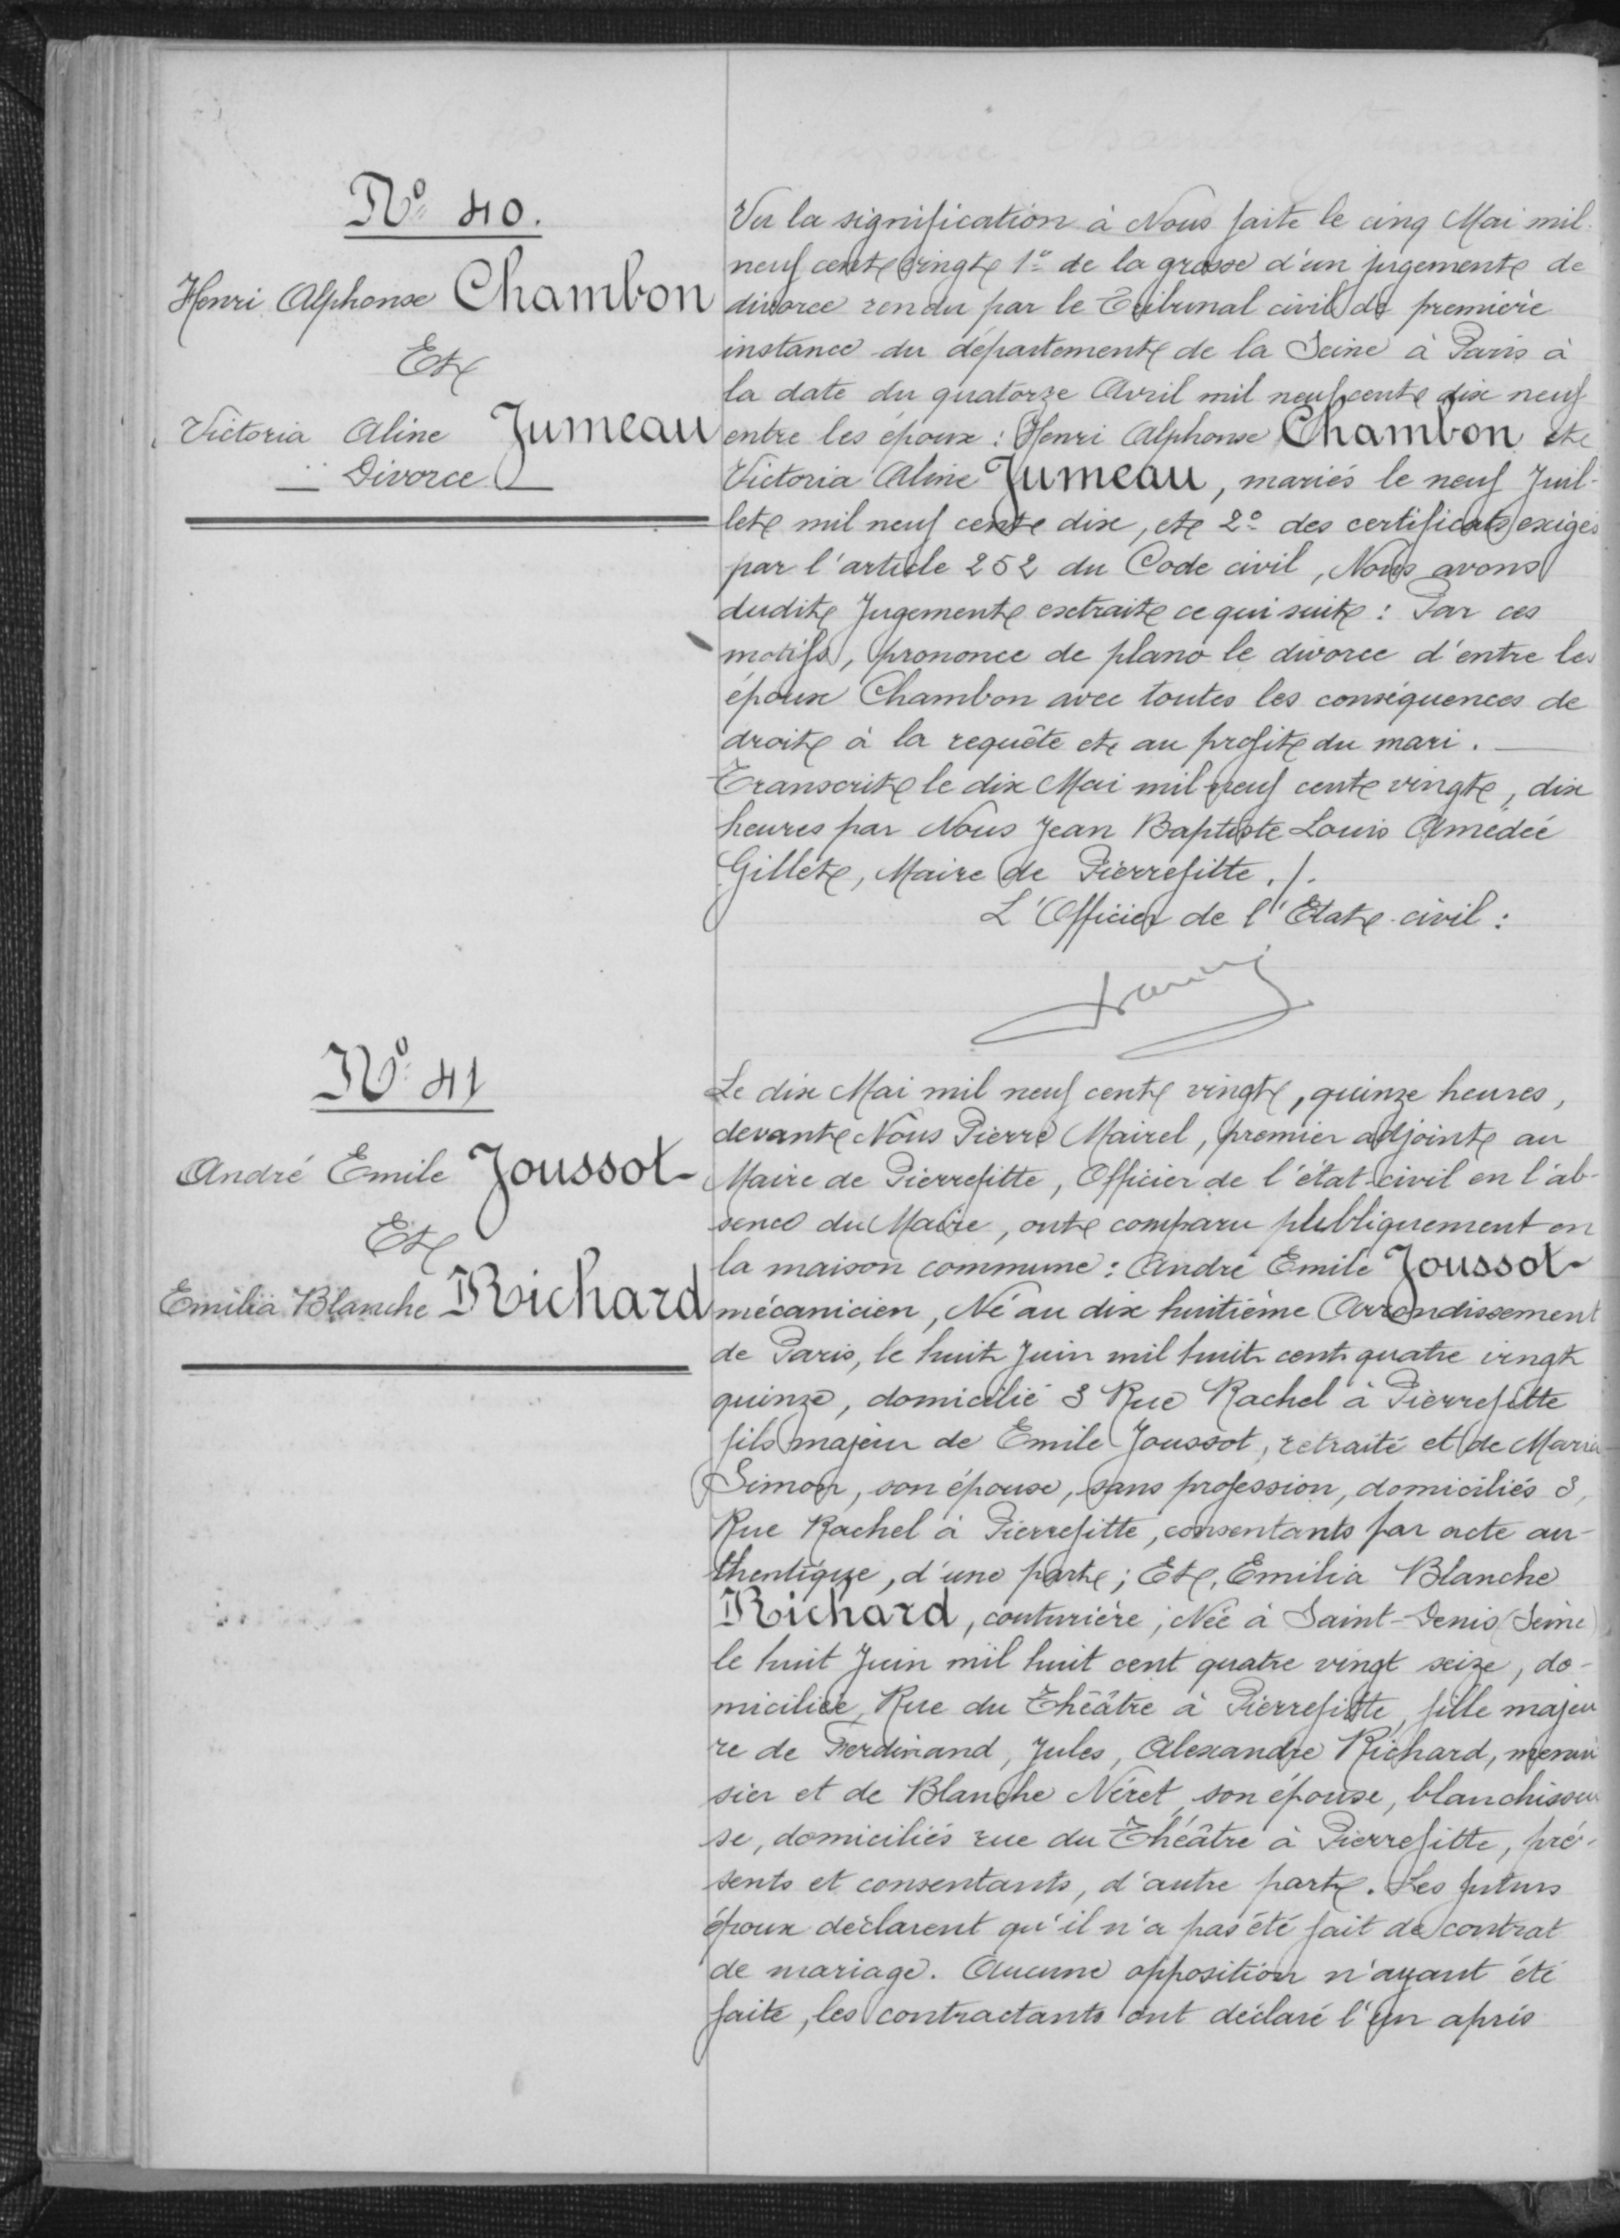N°40
Henri Alphonse Chambon
Et
Victoria Aline Jumeau
Divorce
Vu la signification à Nous faite le cinq Mai mil
neuf cent vingt 1° de la grosse d'un jugement de
divorce rendu par le Tribunal civil de première
instance du département de la Seine à Paris à
la date du quatorze Avril mil neuf cent dix neuf
entre les époux: Henri Alphonse Chambon et
Victoria Aline Jumeau, mariés le neuf Juil-
let mil neuf cent dix, et 2° des certificats exigé
par l'article 252 du Code civil, Nous avons
dudit Jugement extrait ce qui suit: Par ces
motifs, prononce de plano le divorce d'entre les
époux Chambon avec toutes les conséquences de
droit à la requête et au profit du mari.
Transcrit le dix Mai mil neuf cent vingt, dix
heures par Nous Jean Baptiste Louis Amedée
Gillette, Maire de Pierrefitte ./.
L'Officier de l'Etat civil:
N°41
André Emile Joussot
Et
Emilia Blanche Richard
Le dix Mai mil neuf cent vingt, quinze heures,
devant Nous Pierre Mairel, premier adjoint au
Maire de Pierrefitte, Officier de l'état civil en l'ab-
sence du Maie, ont comparu publiquement en
la maison commune: André Emile Joussot
mécanicien, Né au dix huitième Arrondissement
de Paris, le huit Juin mil huit quatre vingt
quinze, domicilié 3 Rue Rachel à Pierrefitte
fils majeur de Emile Joussot, retraité et de Maria
Simon, son épouse, sans profession, domiciliés 3
Rue Rachel à Pierrefitte, consentants par acte au-
thentique, d'une part; Et, Emilia Blanche
Richard, couturière, Ne à Saint-Denis (Seine)
le huit Juin mil huit cent quatre vingt seize, do-
miciliée, Rue du Théâtre à Pierrefitte, fille majeu
re de Ferdinand, Jules, Alexandre Richard, menui
sier et de Blanche Niret, son épouse, blanchisseu
se, domiciliés rue du Théâtre à Pierrefitte, pré
sents et consentants, d'autre part. Les futurs
époux déclarent qu'il n'a pas été fait de contrat
de mariage. Aucune opposition n'ayant été
faite, les contractants ont déclaré l'un après
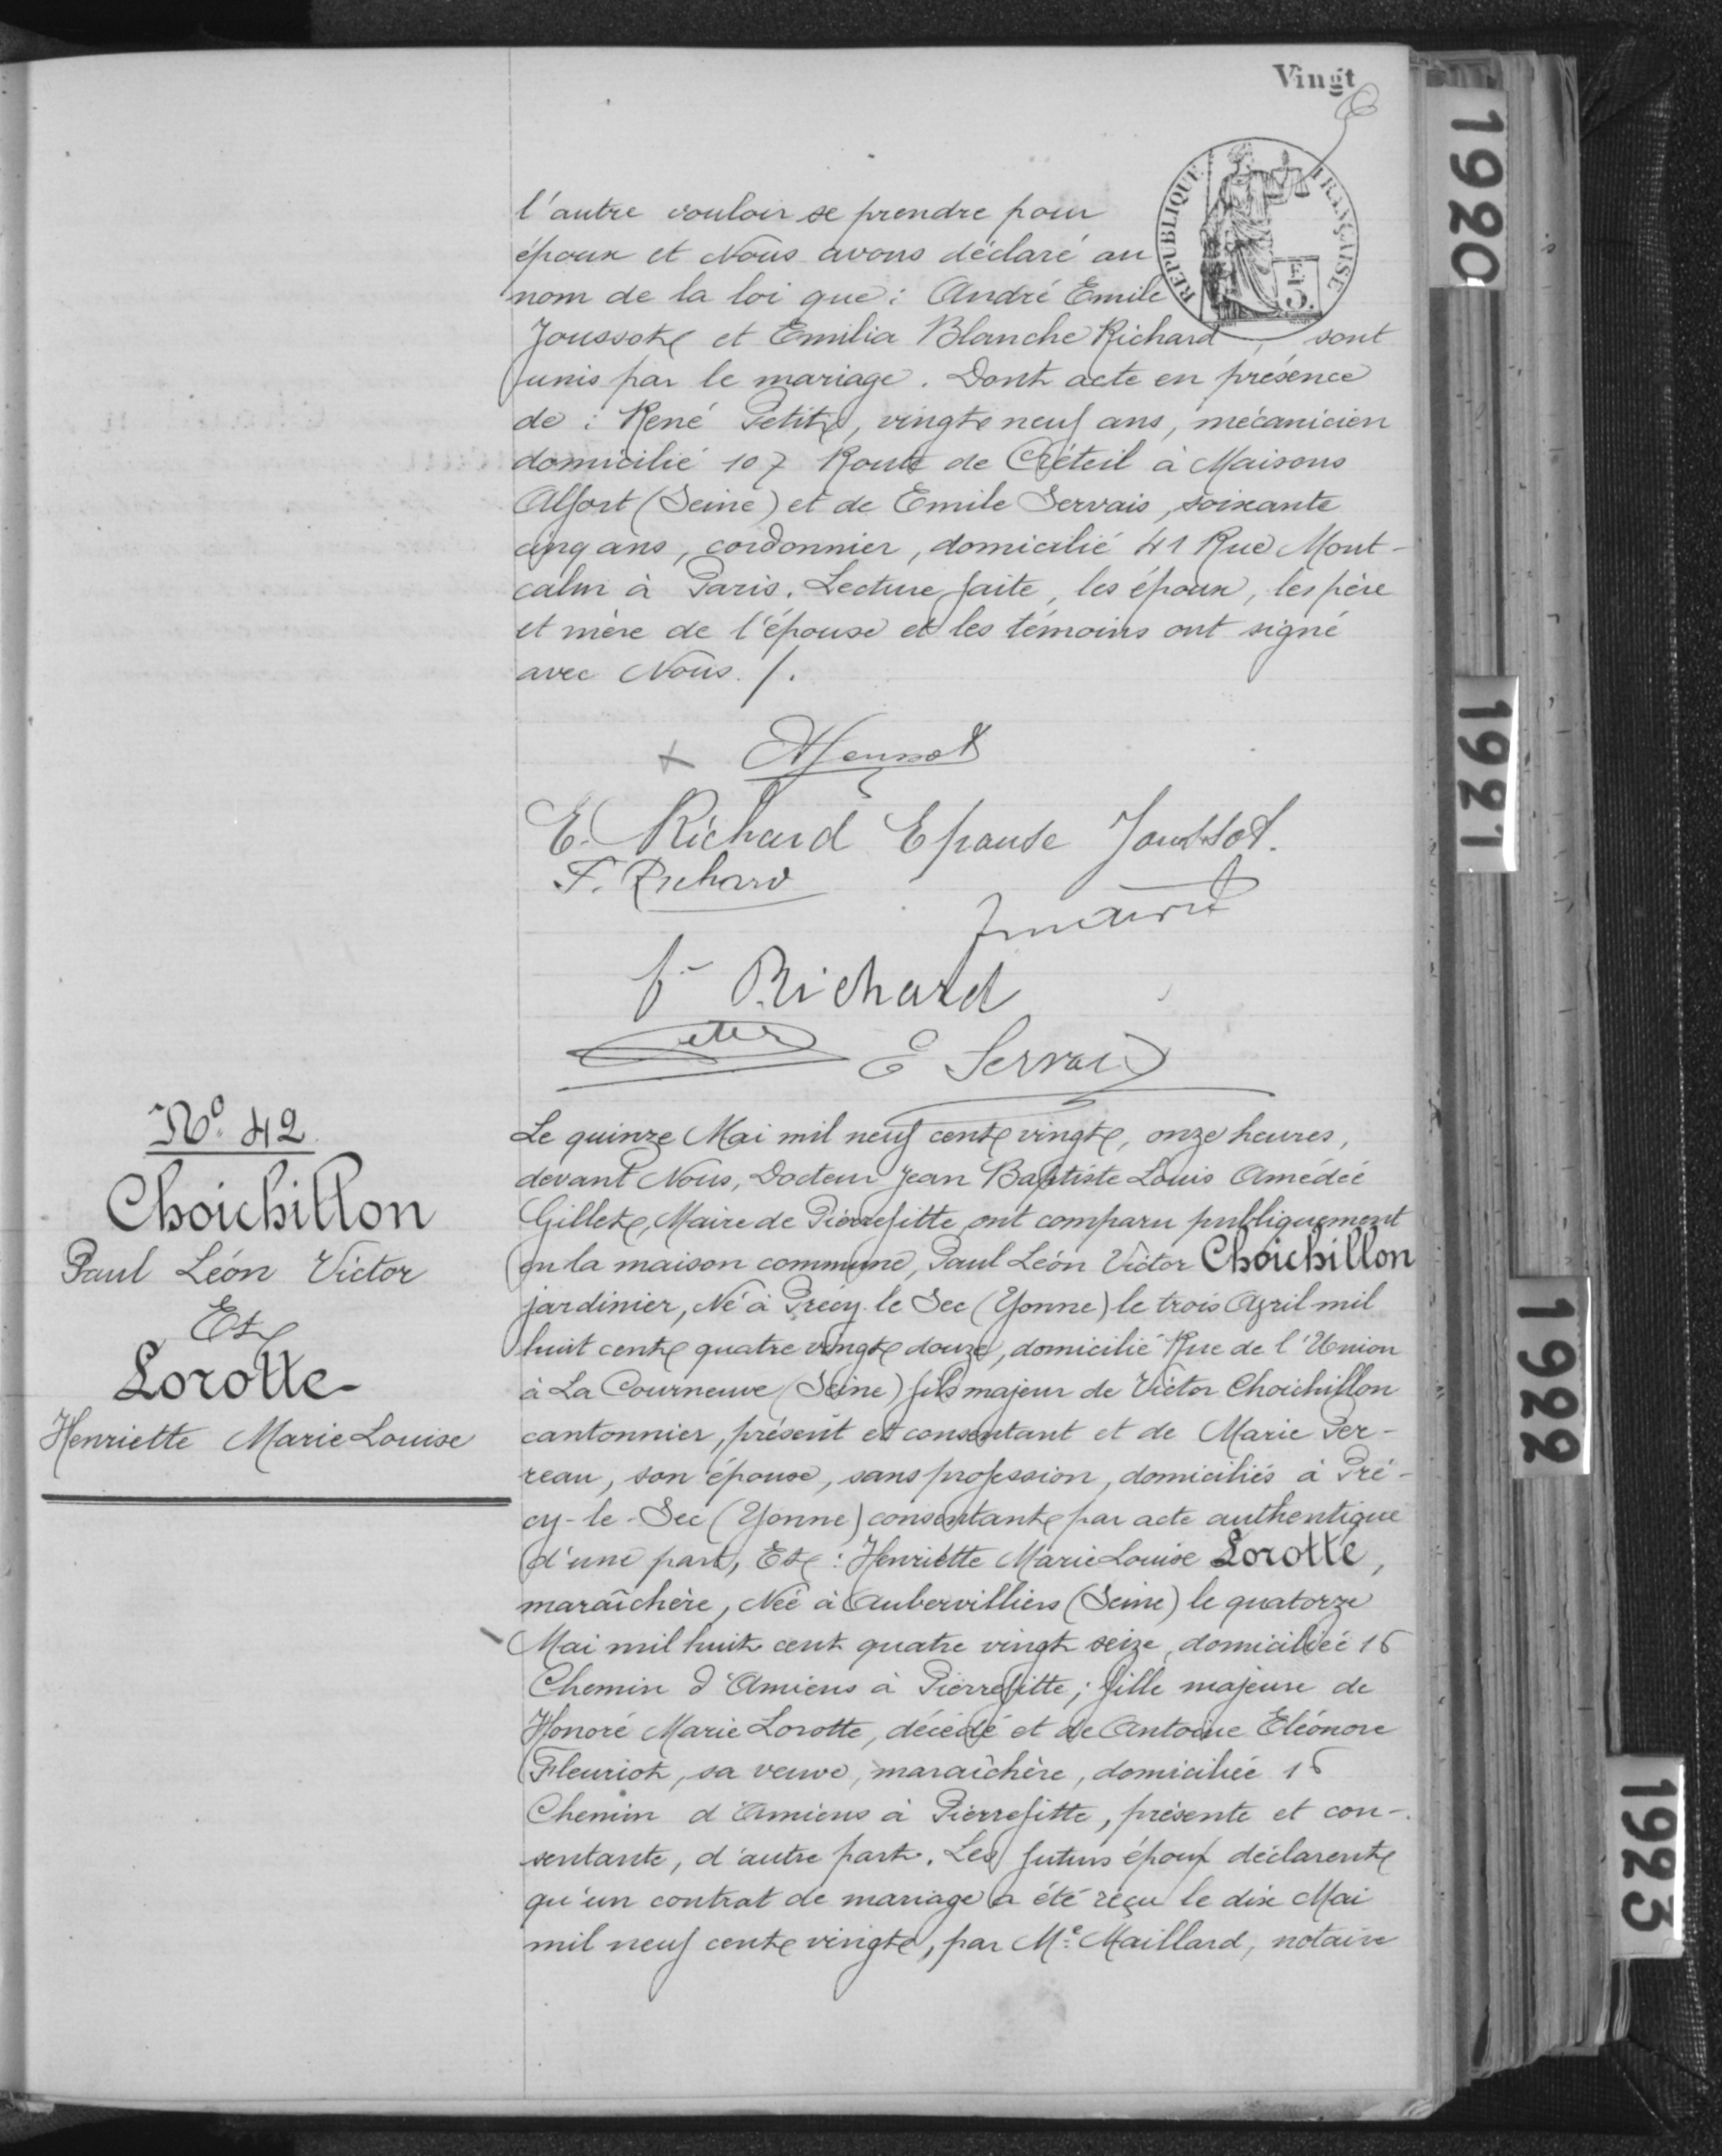l'autre vouloir se prendre pour
époux et Nous avons déclaré au
nom de la loi que: André Emile
Joussot et Emilia Blanche Richard sont
unis par le mariage. Dont acte en présence
de: René Petit, vingt neuf ans, mécanicien
domicilié 107 Route de Créteil à Maisons
Alfort (Seine) et de Emile Servais, soixante
cinq ans, cordonnier, domicilié 41 Rue Mont
calm à Paris, Lecture faite, les époux, les père
et mère de l'épouse et les témoins ont signé
avec Nous ./.
N°42
Choichillon
Paul Léon Victor
Et
Lorotte
Henriette Marie Louise
Le quinze Mai mil neuf cent vingt, onze heures,
devant Nous, Docteur Jean Baptiste Louis Amédée
Gillette, Maire de Pierrefitte, ont comparu publquement
en la maison commune, Paul Léon Victor Choicillon
jardinier, né à Précy le Sec (Yonne) le trois avril mil
huit cent quatre vingt douze, domicilié Rue de l'union
à la Courneuve (Seine) fils majeur de Victor Choichillon
cantonnier, présent et consentant et de Marie Per-
reau, son épouse, sans profession, domiciliés à Pré-
cy-le-Sec (Yonne) consentante par acte authentique
d'une part, Et: Henriette Marie Louise Lorotte,
maraîchère, Née à Aubervilliers (Seine) le quatorze
Mai mil huit cent quatre vingt seize, domiciliée 16
Chemin d'Amiens à Pierrefitte; fille majeure de
Honoré Marie Lorotte, décédé et de Antoine Eleonore
Gleuriot, sa veuve, maraichère, domiciliée 16
Chemin d'Amiens à Pierrefitte, présente et con-
sentante, d'autre part. Les futurs époux déclarent
qu'un contrat de mariage a été reçu le dix Mai
mil neuf cent vingt, par M°: Maillard, notaire